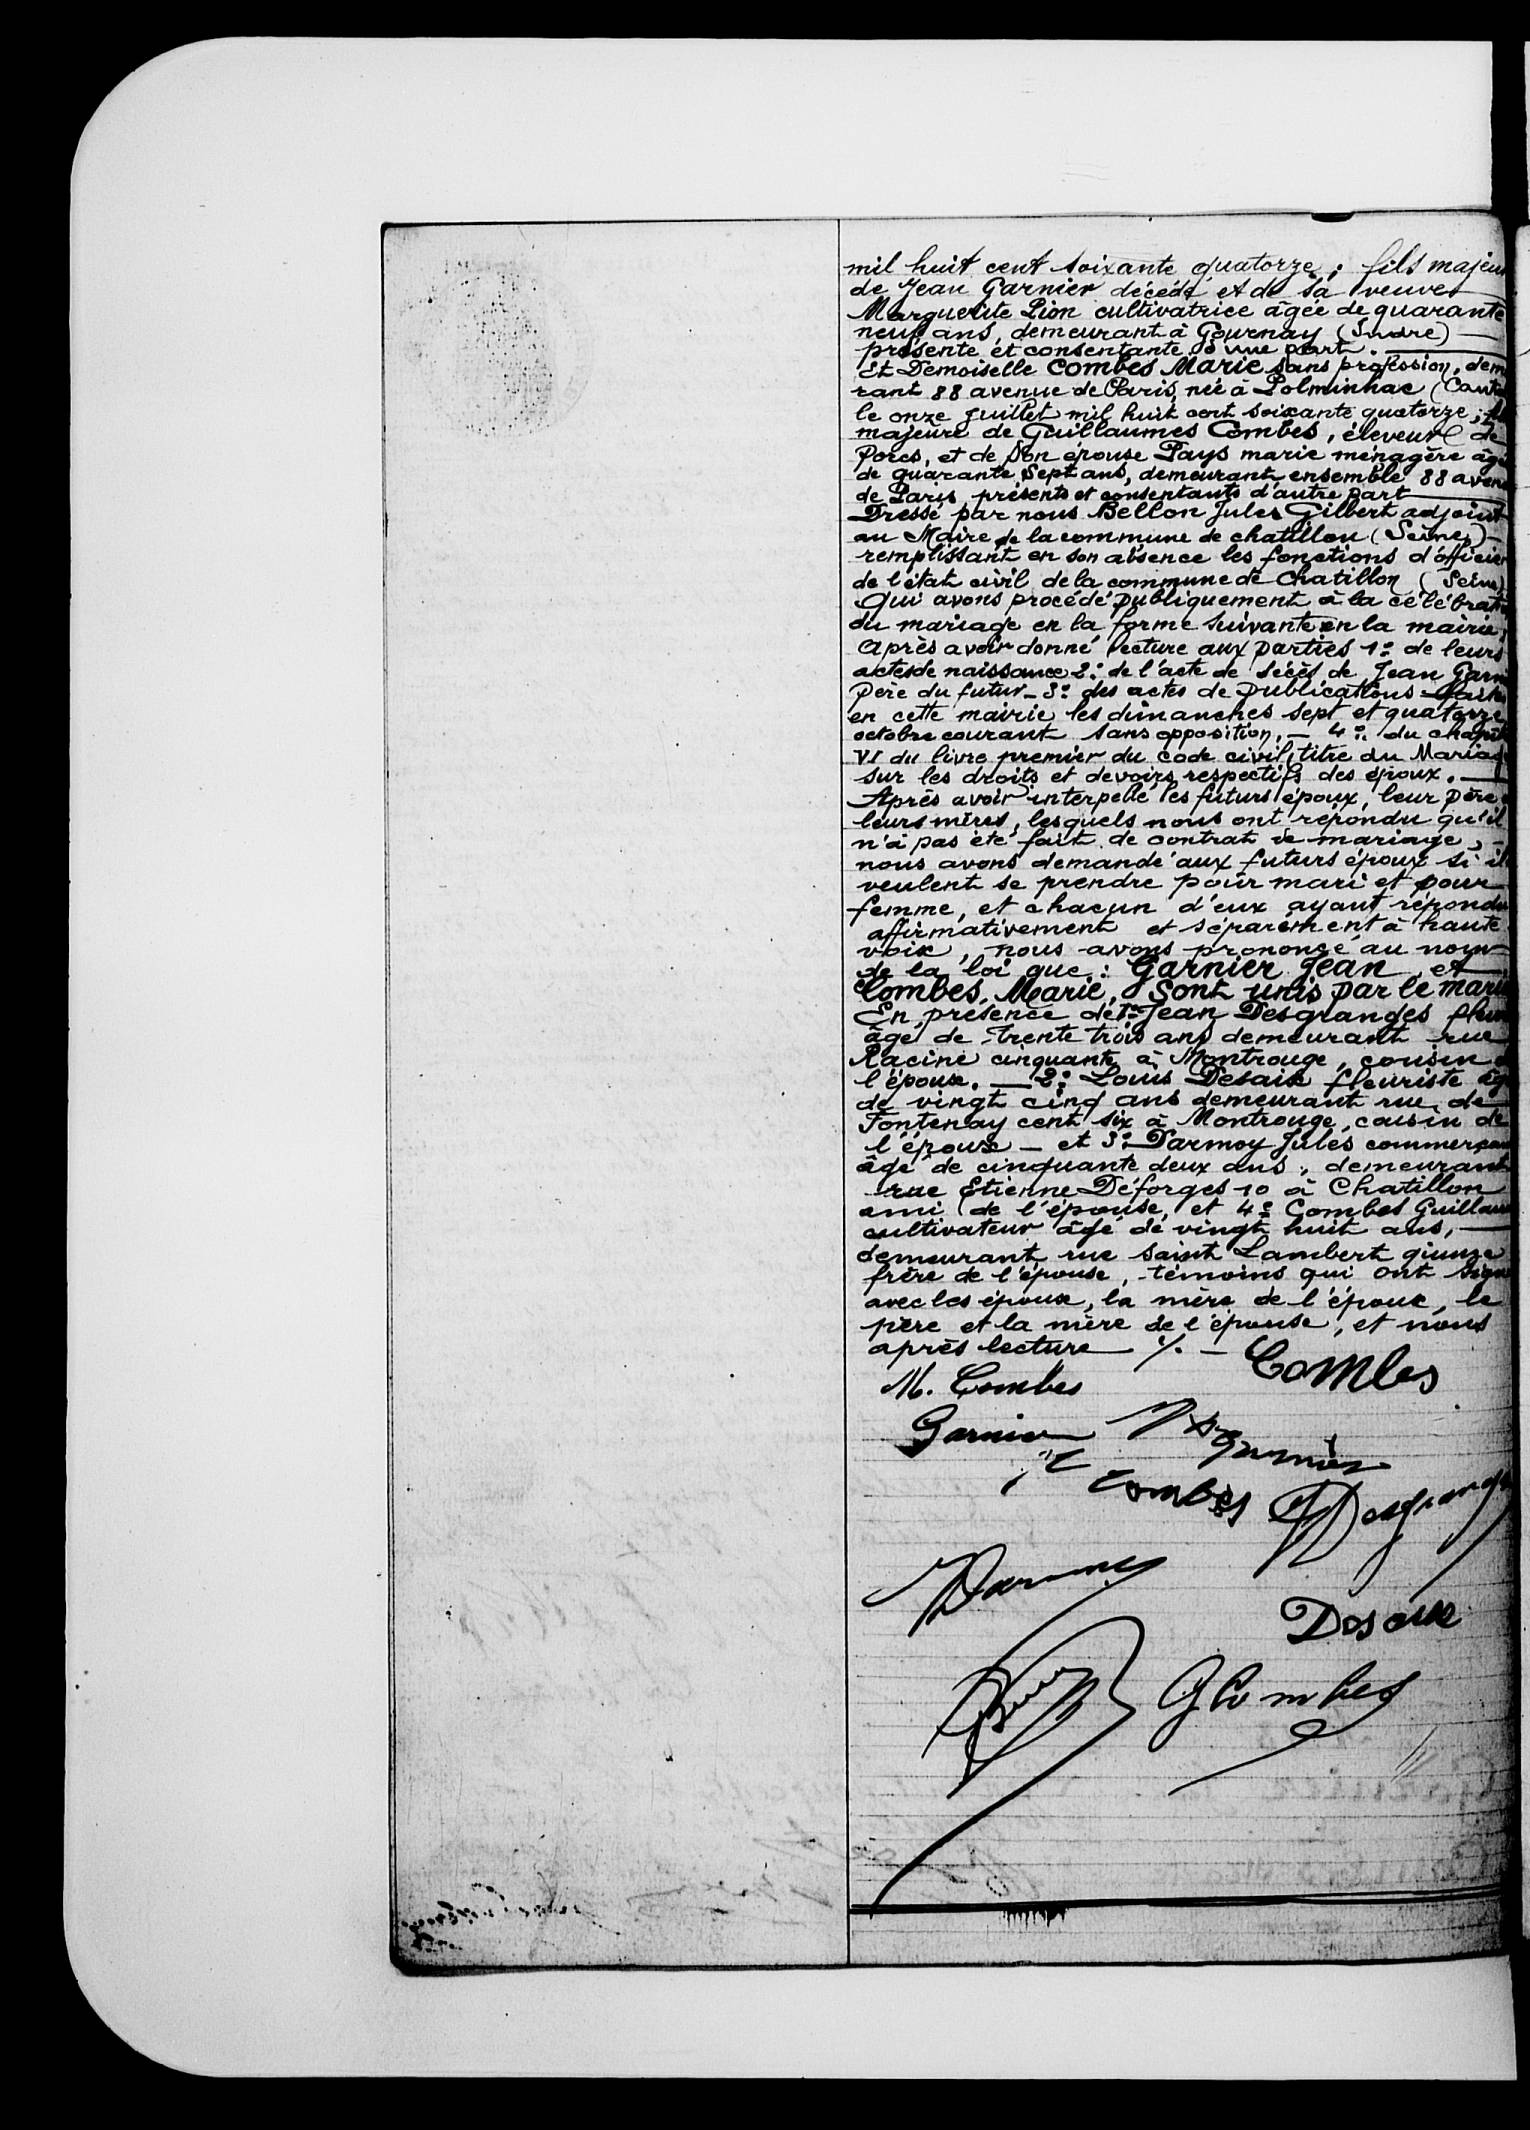mil huit cent soixante quatorze; fils majeur
de Jean Garnier décédé et de sa veuve-
Marguerite Pion cultivatrice âgée de quarante
neuf ans demeurant à Gournay (Indre)-
présente et consentante d'une part.-
Et demoiselle Combes Marie sans profession, dem
rant 88 avenue de Paris née à Polminhac (Cantal
le onze juillet mil huit cent soixante quatorze; f
majeur de Guilaumes Combes, éleveur de
porcs, et de son épouse Pays Marie ménagère âgé
de quarante sept ans, demeurant ensemble 88 avenu
de Paris présents et consentants d'autre part-
Dressé par nous Bellon Jules Gilbert adjoint
au Maire de la commune de chatillon (Seine) -
remplissant en son absence les fonction d'officier
de l'état civil de la commune de Chatillon (Seine)
Qui avons procédé publiquement à la célébrat
du mariage en la forme suivante en la mairie,
Après avoir donné lecture aux parties 1:° de leurs
actes de naissance 2: de l'acte de décès de Jean Garni
père du futur. 3: des actes de publications. Faites
en cette mairie les dimanches sept et quatorze
octobre courant sans opposition, -4°: du chapit
VI du livre premier du code civil titre du Mariage
sur les droits et devoirs respectifs des époux.-
Après avoir interpellé les futurs époux, leur père
leurs mères, lesquels nous ont répondu qu'il
n'a pas été fait de contrat de mariage,-
nous avons demandé aux futurs époux si il
veulent se prendre pour mari et pour
femme, et chacun d'eux ayant répondu
affirmativement et séparément à haute
voix, nous avons prononcé au nom
de la loi que: Garnier Jean et
Combes Marie, sont unis par le mari
En présence de: Jean Desgrandes fleur
agé de trente trois ans demeurant rue
Racine cinquante à Montroux, cousin d
l'épouse.-2°: Louis Desaix fleuriste, âg
de vingt cinq ans demeurant rue de
Fontenay cent six à Montrouge, cousin de
l'époux -et 3° Varnoy Jules commerçant
âgé de cinquante deux ans, demeurant
rue Etienne Déforges 10 à Chatillon
ami de l'épouse et 4: Combes Guillaume
cultivateur âgé de vingt huit ans,
demeurant rue saint Lambert quinze
frère de l'épouse, témoins qui ont signé
avec les époux, la mère de l'époux, le
père et la mère de l'épouse, et nous
après lecture ./. -
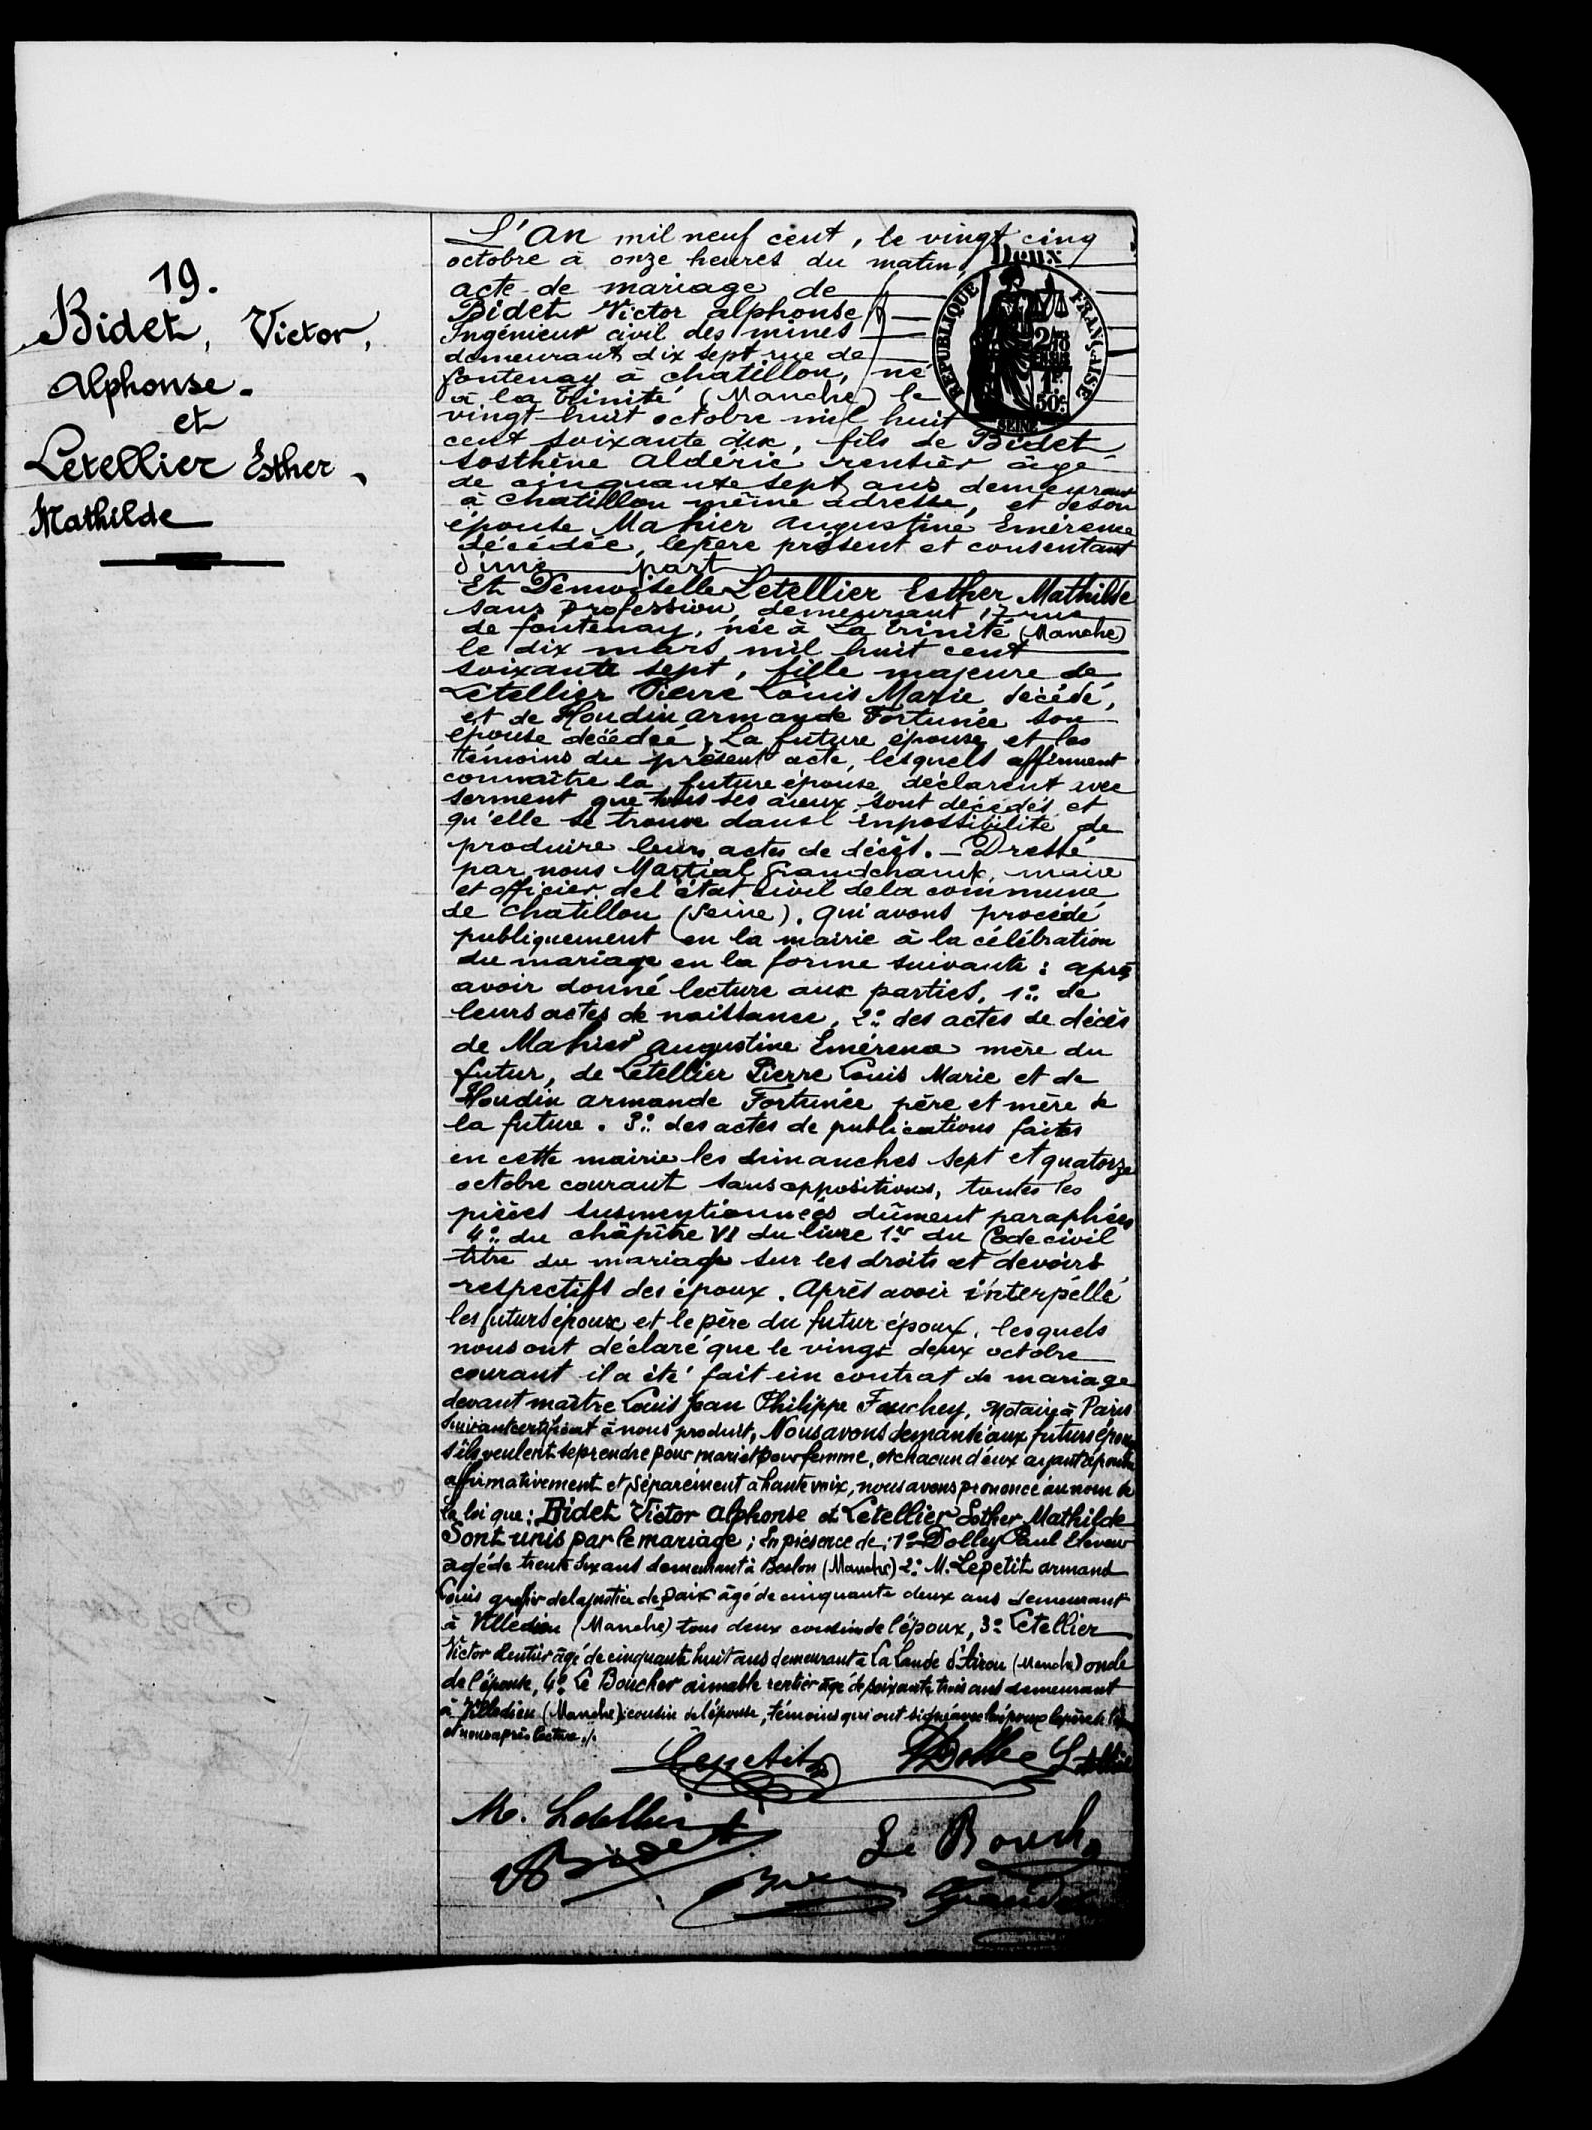19.
Bidet, Victor,
Alphonse.
et
Letellier Ester,
Mathilde
L'an mil neuf cent le vingt cinq
octobre à onze heures du matin,
acte de mariage de Bidet Victor alphonse
ingénieur civil des mines
demeurant dix sept rue de
fontenay à chatillon, né
à la Trinité (Manche) le
vingt huit octobre mil huit
ceux soixante dix, fils de Bidet,
Sosthène Aldérie rentier âgé
de cinquante sept ans demeurant
à chatillon même adresse, et de son
épaule Mahier augustine Emerence
décédée, le père présent et consentant
d'une part-
Et Demoiselle Letellier Esther Mathilde
sans profession demeurant 17 rue
de fontenay, née à La Trinité (Manche)
le dix mars mil hit cent-
soixante sept, fille majeure de
Letellier Pierre Louis Marie décédé,
et de Houdin armande Fortunée son
épouse décédée, la future épouse et les
témoins du présent acte, lesquels affirment
connaître la future épouse déclarent avec
serment que tous les aieux sont décédés et
qu'elle se trouve dans l'impossibilité de
produire leurs actes de décès. -Dréssé-
par nous Martial Grandchant, maire
et officier de l'état civil de la commune
de Chatillon (Seine) qu'avons procédé
publiquement en la mairie à la célébration
du mariage en la forme suivante: après
avoir donné lecture aux parties, 1° de
leurs actes de naissance, 2° des actes de décès
de Mahier augustine Emérence mère du
futur, de Letellier Pierre Louis Marie et de
Houdin armande Fortunée père et mère de
la future. 3° des actes de publications faites
en cette mairie les dimanches sept et quatorze
octobre courant sans oppositions, toutes les
pièces susmentionnéess dûment paraphées
4° du châpître VI du livre 1er du Code civil
titre du mariage sur les droits et devoirs
respectifs des époux. Après avoir interpellé
les futurs époux et le père du futur époux, lequels
nous ont déclaré que le vingt deux octobre
courant il a été fait un contrat de mariage
devant maître Louis Jean Philippe Fauchey, Notaire à Paris
suivant certificat à nous produit, Nous avons demandé aux futurs époux
s'ils veulent se prendre pour mari et pour femme, et chacun d'eux ayant répondu
affirmativement et séparément à haute voix, nous avons prononcé au nom de
la loi que: Bidet Victor Alphonse et Letellier Esther Mathilde
sont unis par le mariage; En présence de: 1° Dolly Paul eleveur
agé de trente six ans demeurant à Berlon (Manche) 2° M. Lepetit armand
Louis greffier de la justice de paix âgé de cinquante deux ans demeurant
à Villedieu (Manche) tous deux cousin de l'époux, 3° Letellier
Victor Rentier âgé de cinquante huit ans demeurant à la Lande d'Airou (Manche) oncle
de l'épouse, 4° le Boucher aimable rentier âgé de soixante trois ans demeurant
à Villedieu (Manche) cousin de l'épouse, témoins qui ont signé avec les époux, le père de l'époux
et nous après lecture ./.